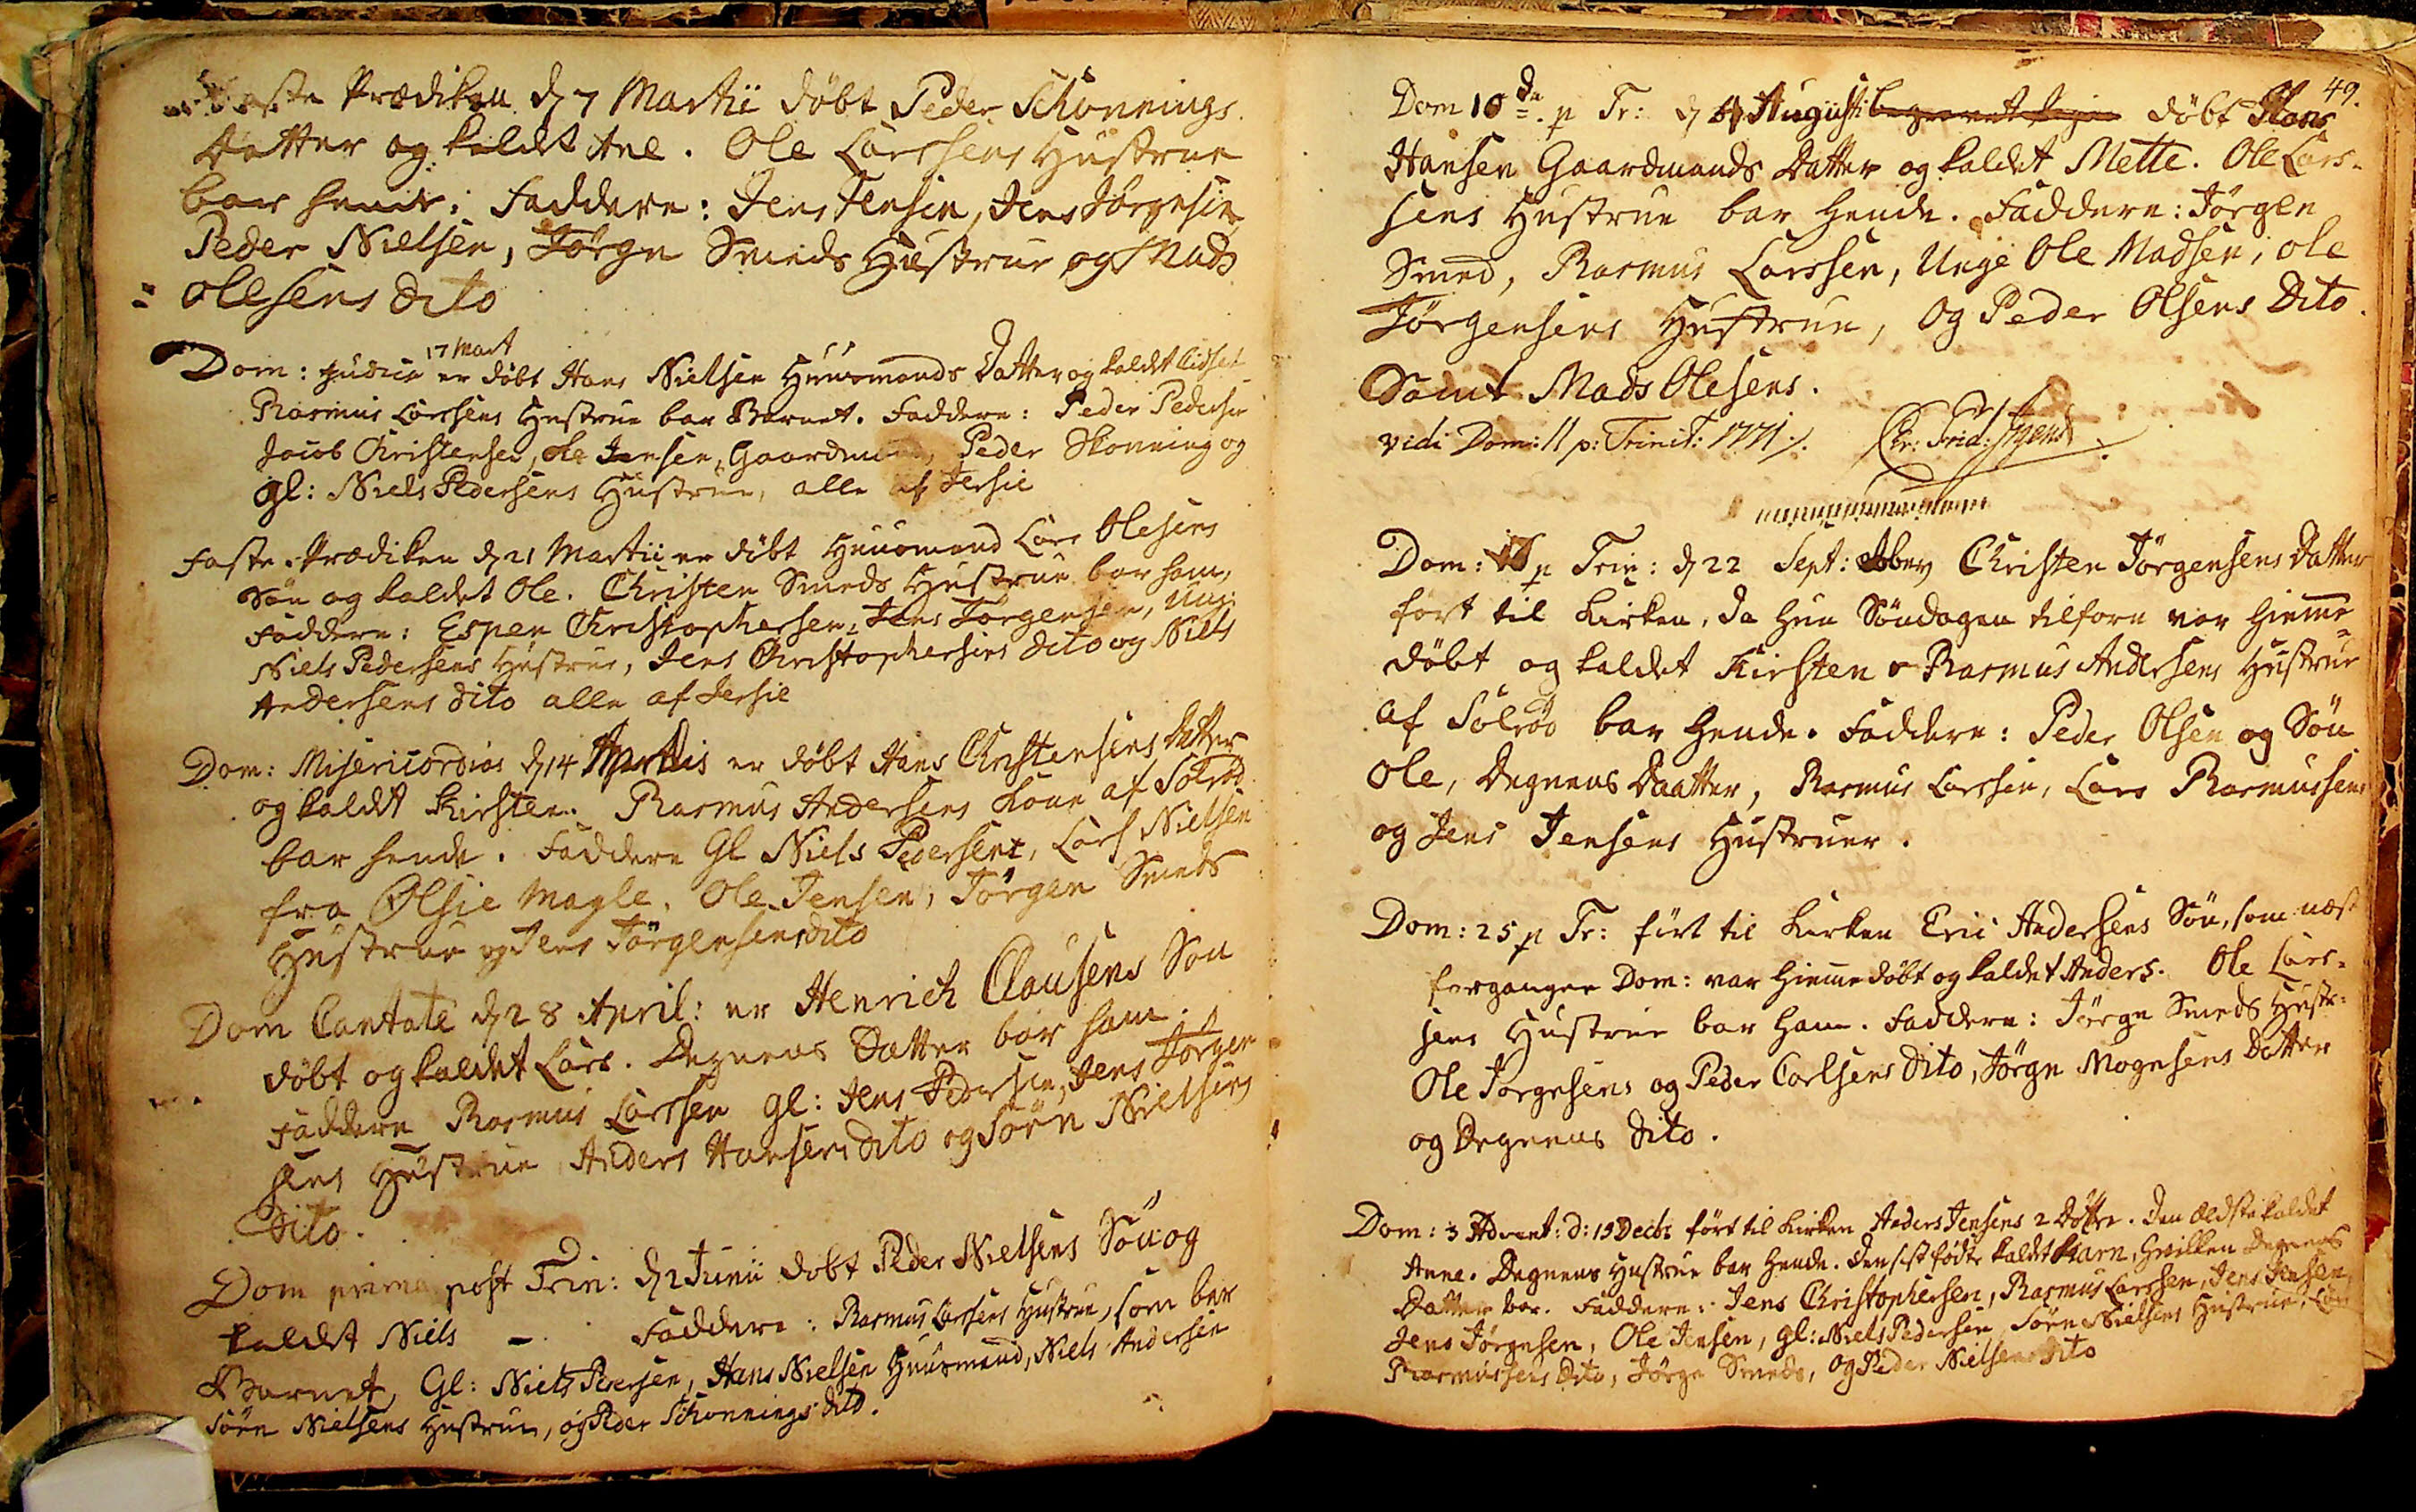Faste Prædiken d 7 Martii døbt Peder Schonnings
Datter og kaldt Ane. Ole Larssens Hustrue
bar hende. Faddere: Jens Jensen, Jens Jørgensen,
Peder Nielsen, Jørgn Smids Hustrue og Mads
Olsens dito
17 Mart
Dom: Judica er døbt Hans Nielsen Huusmands Datter og kaldt Cidsel
Rasmus Larssens Hustrue bar Barnet. Faddere: Peder Pedersen
Jacob Christensen, Ole Jensen, Gaardmand, Peder Skonning og
gl: Niels Pedersens Hustrue, alle af Jersie
Faste-Prædiken d 21 Martii er døbt Huusmand Lars Olesens
Søn og kaldet Ole. Christen Smeds Hustrue bar ham,
Faddere: Espen Christophersen, Jens Jørgensen, Ung:
Niels Pedersens Hustrue, Jens Christophersens dito og Niels
Andersens dito alle af Jersie
Dom: Misericordias d 14 Aprilis er døbt Hans Christensens Datter
og kaldt Kirsten. Rasmus Andersens Kone af Solrød
bar hende. Faddere Gl Niels Pedersens, Lars Nielsen
fra Ølsie Magle. Ole Jensen, Jørgen Smeds
Hustrue og Jens Jørgensens dito
Dom Cantate d 28 April: er Henrich Clausens Søn
døbt og kaldet Lars. Degnens Datter bar ham.
Faddere Rasmus Larssen gl: Jens Pedersen, Jens Jørgen
sens Hustrue, Anders Hansens dito og Sørn Nielsens
dito.
Dom prima post Trin: d 2 Junii døbt Peder Nielsens Søn og
kaldet Niels
Faddere: Rasmus Larssens Hustrue, som bar
Barnet, Gl: Niels Pedersen, Hans Nielsen Huusmand, Niels Andersen
Sørn Nielsens Hustrue, og Peder Schonnings dito.
49.
Dom 10de. p Tr: d 4 Augusti begravet Pigen døbt Hans
Hansen Gaardmands Datter og kaldet Mette. Ole Lars
sens Hustrue bar hende. Faddere: Jørgen
Smed, Rasmus Larssen, unge Ole Madsen; Ole
Jørgensens Hustrue, Og Peder Olsens Dito.
Samt Mads Olesens.
Vidi Dom: 11 p: Trinit: 1771 ./. 
Chr: Frid: Irgens
Dom: 17 p Trin: d 22 Sept: ##bev Christen Jørgensens Datter
ført til Kirken, da hun Søndagen tilforn var hiemme
døbt og kaldet Kirsten. Rasmus Andersens Hustrue
af Solrød bar hende. Faddere: Peder Olsen og Søn
Ole, Degnens Daatter, Rasmus Larssen, Lars Rasmussens
og Jens Jensens Hustruer.
Dom: 25 p Tr: ført til Kirken Eric Andersens Søn, som næst
forgangne Dom: var hiemmedøbt og kaldet Anders. Ole Lars¬
sens Hustrue bar ham. Faddere: Jørgen Smeds Hustr:
Ole Jørgnsens og Peder Carlsens dito, Jørgn Mognsens Datter
og Degnens dito.
Dom: 3 Advent: d: 15 Decbr ført til Kirken Anders Jensens 2 døttre. Den ældste kaldet
Anne. Degnens Hustrue bar hende. Den sist fødte kaldt Karn, hvilken Degnens
Datter bar. Faddere: Jens Christophersen, Rasmus Larssen, Jens Jensen,
Jens Jørgnsen, Ole Jensen, gl: Niels Pedersen, Sørn Nielsens Hustrue, Lars
Rasmussens dito, Jørgen Smeds, og Peder Nielsen dito
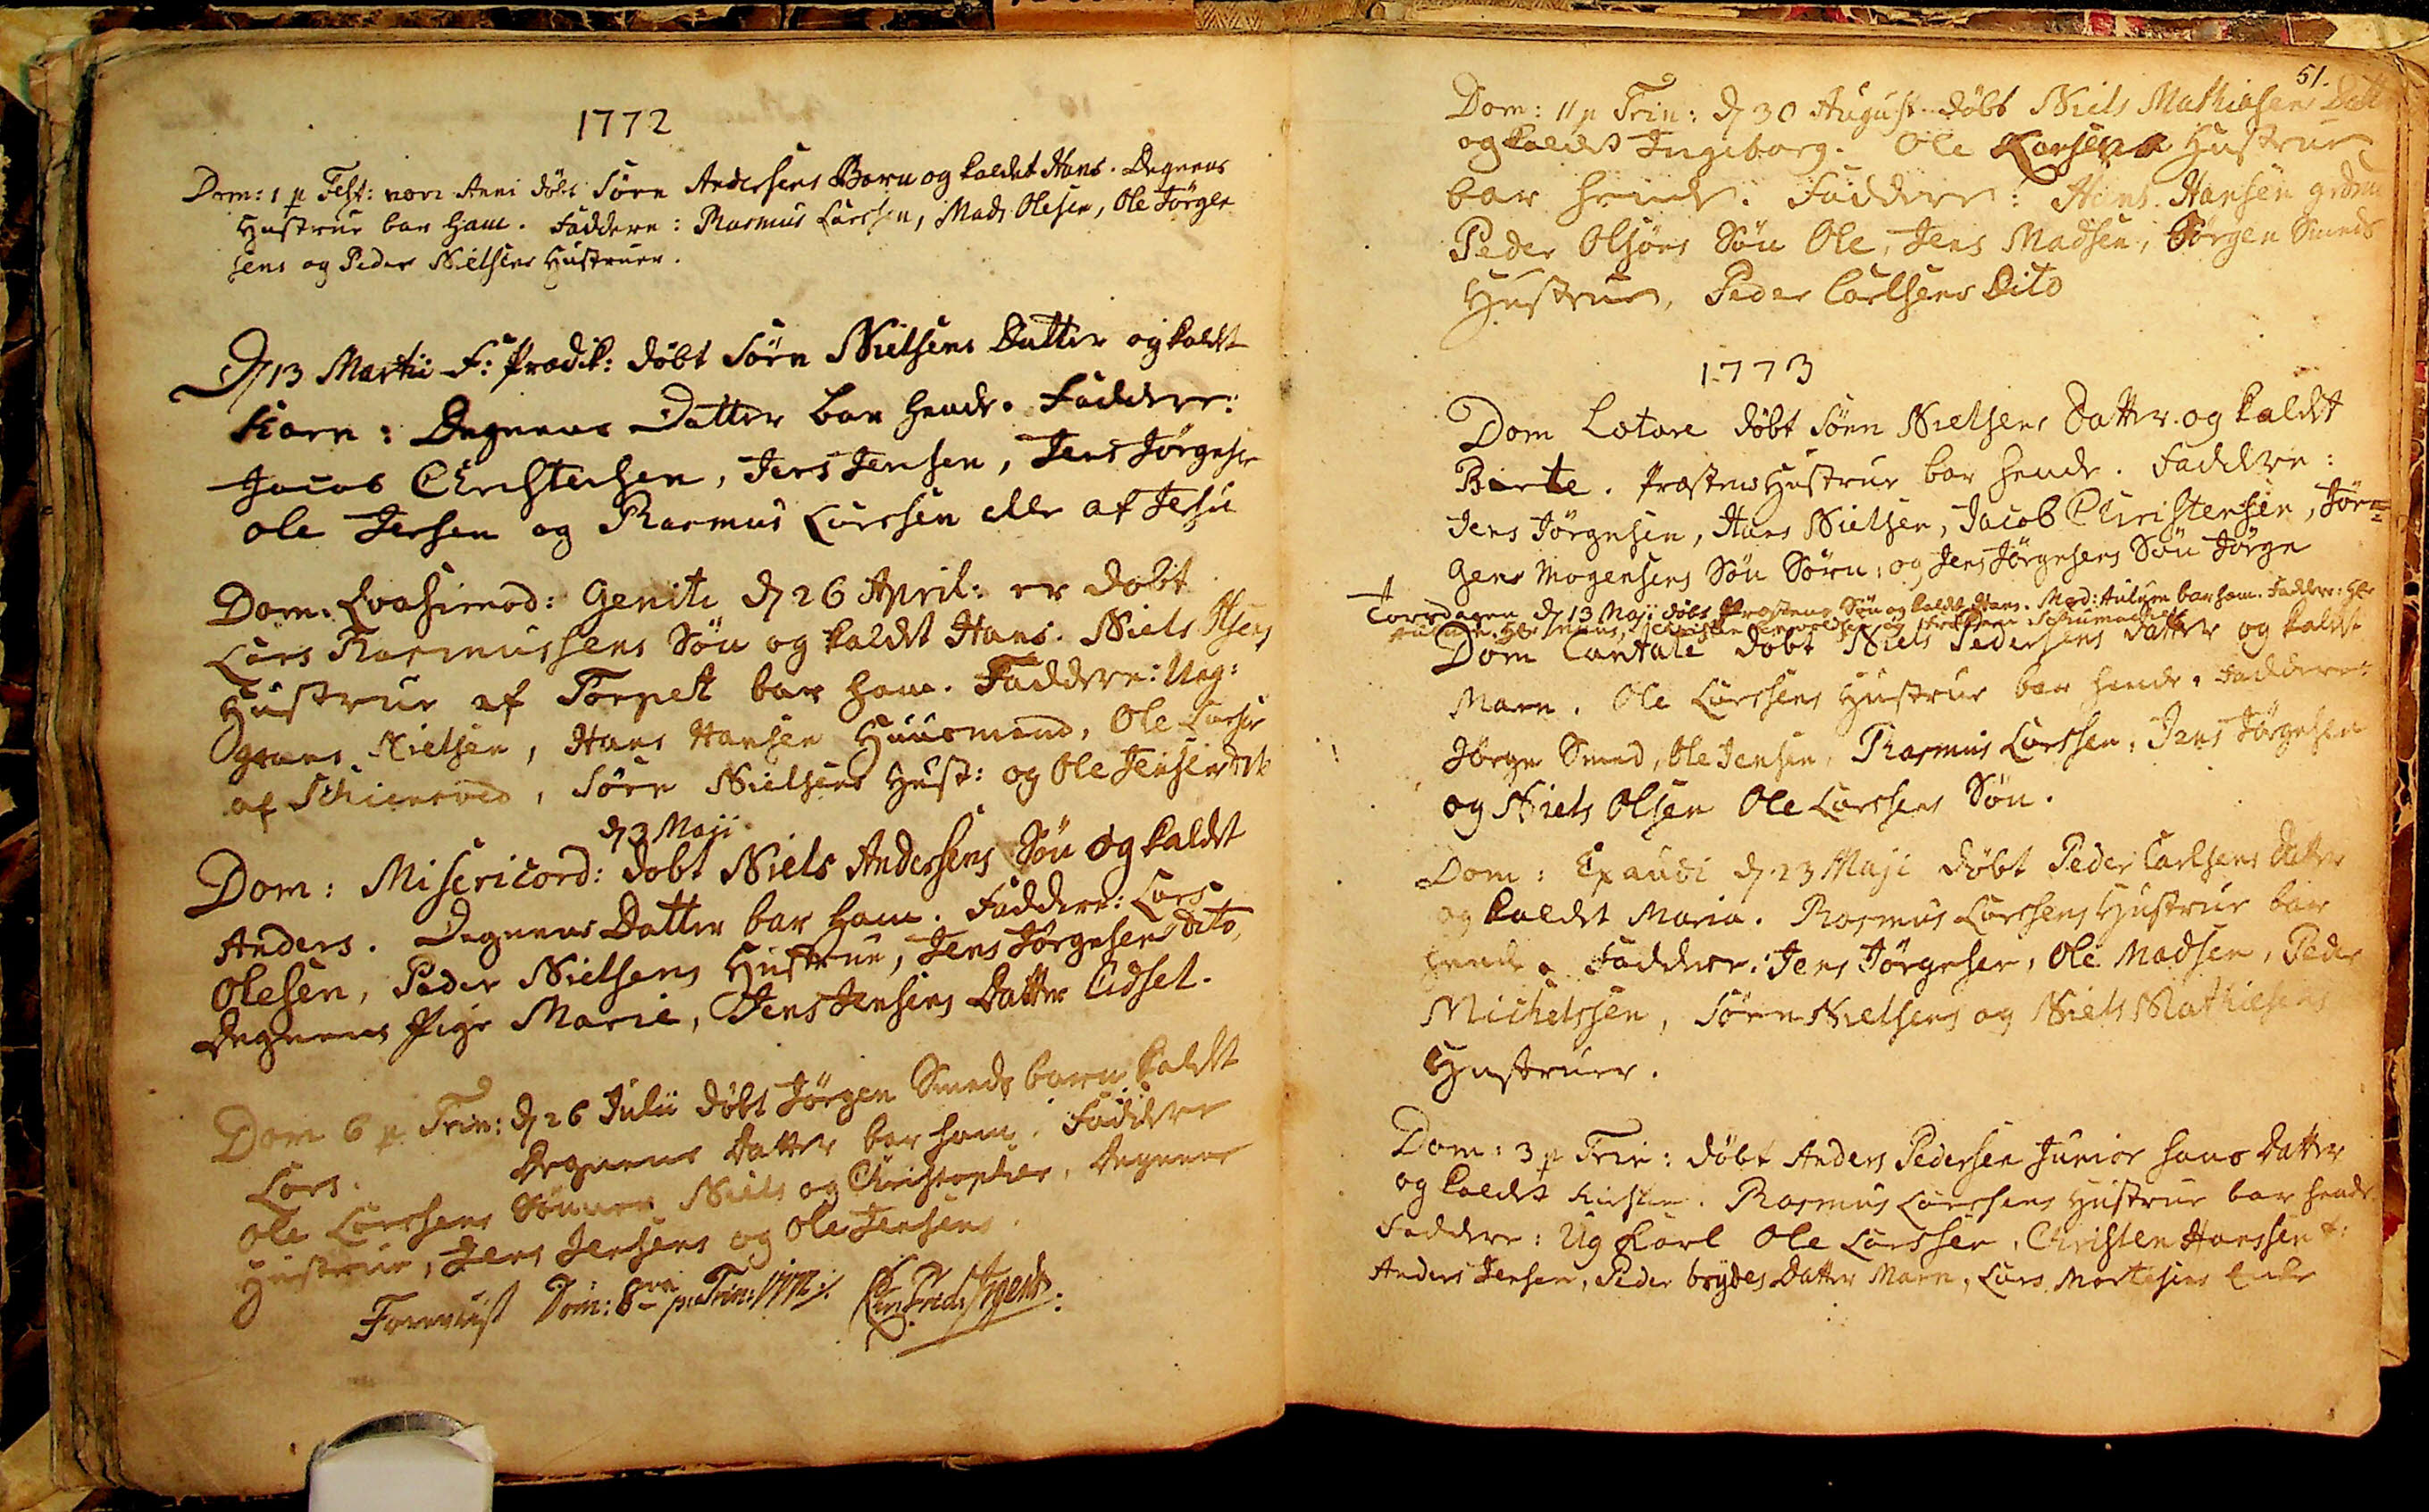1772
Dom:1 p Fest: novi Anni døbt Sørn Andersens Barn og kaldet Hans. Degnens
Hustrue bar ham. Faddere: Rasmus Larsen, Mads Olesen, Ole Jørgen
sens og Peder Nielsens Hustruer.
D 13 Martii F: Prædik: døbt Sørn Nielsens Datter og kaldt
Karn: Degnens Datter bar hende. Faddere:
Jacob Christensen, Jens Jensen, Jens Jørgnsen
Ole Jensen og Rasmus Larsen alle af Jersie
Dom. Qvasimod: Geniti d 26 April: er døbt
Lars Rasmussens Søn og kaldt Hans. Niels Olsens
Hustrue af Torpet bar ham. Faddere: Ung:
Hans Nielsen, Hans Hansen Huusmand, Ole Larsen
af Schiensved, Sørn Nielsens Hust: og Ole Jensens dito
d 3 Maji
Dom: Misericord: døbt Niels Andersens Søn og kaldet
Anders. Degnens Datter bar ham. Faddere: Lars
Olesen, Peder Nielsens Hustrue, Jens Jørgensens dito
Degnens Pige Marie, Jens Jensens Datter Cidsel.
Dom 6 p Trin: d 26 Julii døbt Jørgen Smeds Barn kaldt
Lars.     
Degnens Datter bar ham. Faddere
Ole Larssens Sønner Niels og Christopher, Degnens
Hustrue, Jens Jensens og Ole Jensens.
Foreviist Dom: 8va p: Trin: 1772./.
Chr: Frid: Irgens.
51.
Dom: 11 p Trin: d 30 August døbt Niels Mathiasens Datt
og kaldet Ingeborg. Ole Larsens Hustrue
bar hende. Faddere: Hans Hansen grdmd
Peder Olsøns Søn Ole, Jens Madsen, Jørgen Smeds
Hustrue, Peder Carlsens Dito
1773
Dom Lætare døbt Sørn Nielsens Datter og kaldet                
Birte. Præstens Hustrue bar hende. Faddere:
Jens Jørgensen, Hans Nielsen, Jacob Christensen, Jør¬
gen Mogensens Søn Sørn, og Jens Jørgensens Søn Jørgn
Torsdagen d 13 Maii døbt Præstens Søn og kaldt Hans . Mad: Aulum bar ham. Faddere: Hr
Aulum. Hr ##, Christen Enevoldsen og Frøken Schumacher
Dom Cantate døbt Niels Pedersens Datter og kaldt
Marn. Ole Larssens Hustrue bar hende. Faddere:
Jørgn Smed, Ole Jensen, Rasmus Larssen, Jens Jørgnsen
og Niels Olsen Ole Larssens Søn.
Dom: Exaudi d 23 Maji døbt Peder Carlsens Datter
og kaldet Maria. Rasmus Larssens Hustrue bar
hende. Faddere: Jens Jørgnsen, Ole Madsen, Peder
Michelssen, Sørn Nielsens og Niels Mathiesens
Hustruer.
Dom: 3 p Trin: døbt Anders Pedersen Junior hans Datter
og kaldet Kirsten. Rasmus Larssens Hustrue bar hende.
Faddere: Ug Karl Ole Larssen, Christen Hanssen t:
Anders Jensen, Peder Brydes Datter Marn, Lars Mortesens Enke